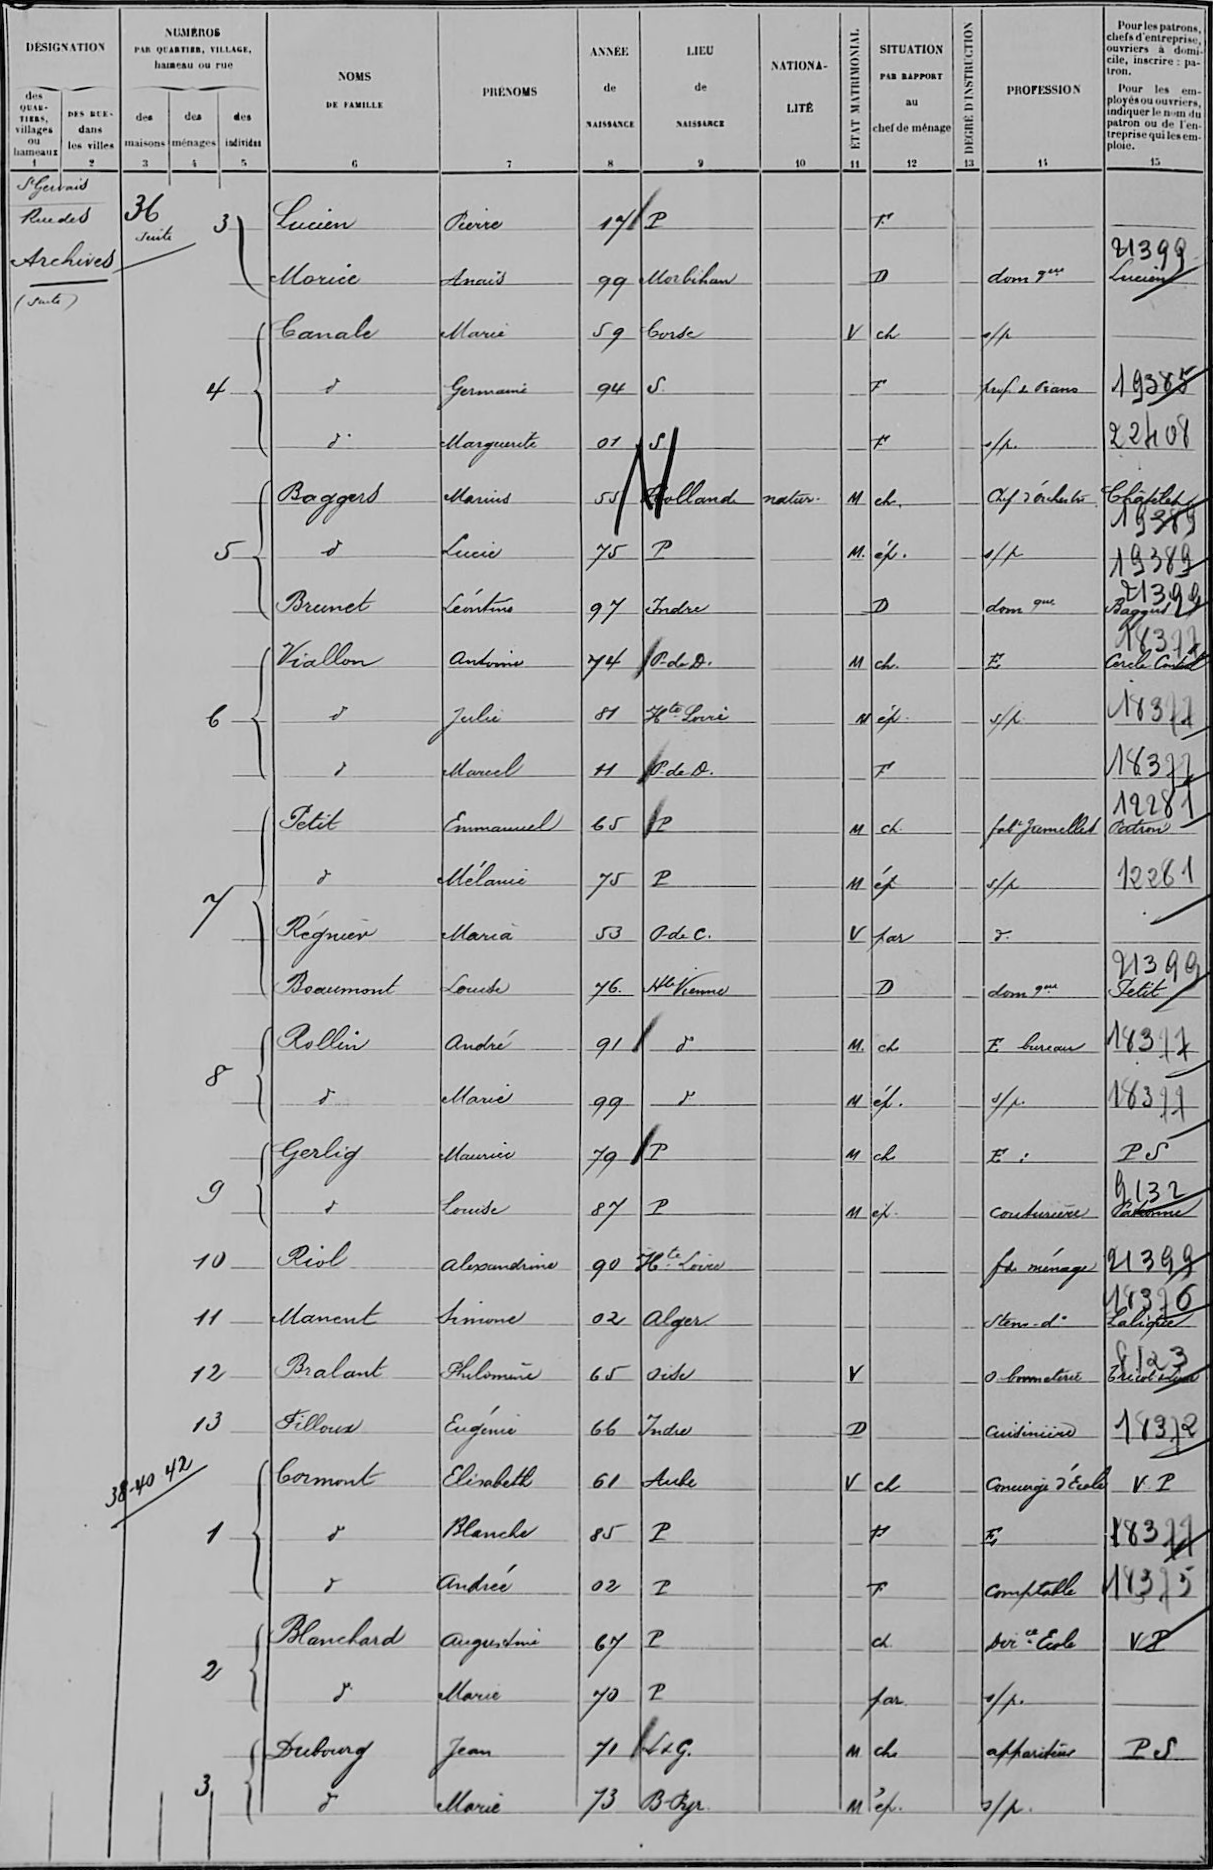Lucien/Pierre/17/P/¤/¤/F/¤/¤/¤
Morin/Anais/99/Morbihan/¤/¤/D/¤/domque/Lucien?21399
Canale/Marie/59/Corse/¤/V/ch/¤/s:p/¤
d/germaine/94/S /¤/¤/F/¤/prof de piano/19385
d/Marguerite/01/S/¤/¤/F/¤/s:p/22408
Baggers/Marius/55/Hollande/natur /M/ch/¤/Chef dorchestre/Châtelet!19389
d/Lucie/75/P/¤/M /ép/¤/s:p/19389
Brunet/léontine/97/Indre/¤/¤/D/¤/domque/Baggers?21399
Viallon/antoine/74/P de D /¤/M/ch /¤/E/Cercle Courtal?18377
d/Julie/81/Hte Loire/¤/M/ép/¤/s:p/18377
d/Marcel/11/P de D /¤/¤/F/¤/¤/18377
Petit/Emmanuel/65/P/¤/M/ch /¤/fabt jumelles/Patron12281
d/Mélanie/75/P/¤/M/ép/¤/s:p/12281
Régnier/Maria/53/P de C /¤/V/par/¤/d /¤
Beaumont/Louise/76/Hte Vienne/¤/¤/D/¤/domque/Petit?21399
Rollin/André/91/d/¤/M/ch/¤/E bureau/18377
d/Marie/99/d/¤/M/ép /¤/s:p/18377
Gerlig/Maurice/79/P/¤/M/ch/¤/E/P S
d/Louise/87/P/¤/M/ép/¤/couturière/Patronne9132
Riol/Alexandrine/90/Hte Loire/¤/¤/¤/¤/F de ménage/21399
Manent/Simone/02/Alger/¤/¤/¤/¤/Steno d°/Lalique?18376
Bralant/Philomène/65/oise/¤/V/¤/¤/o bonneterie/tricot adim?8123
Filloux/Eugénie/66/Indre/¤/D/¤/¤/cuisinière/18372
Cormont/Elisabeth/61/Aube/¤/V/Ch/¤/Concierge d'école/V P
d/Blanche/85/P/¤/¤/F/¤/E/18377
d/Andrée/02/P/¤/¤/F/¤/Comptable/18375
Blanchard/Augustine/67/P/¤/¤/ch/¤/Dirce Ecole/VP
d/Marie/80/P/¤/¤/par/¤/s:p/¤
Dubourg/Jean/71/L & G/¤/M/ch /¤/appariteur/PS
d/Marie/73/B Pyr/¤/M/ép/¤/s:p/¤
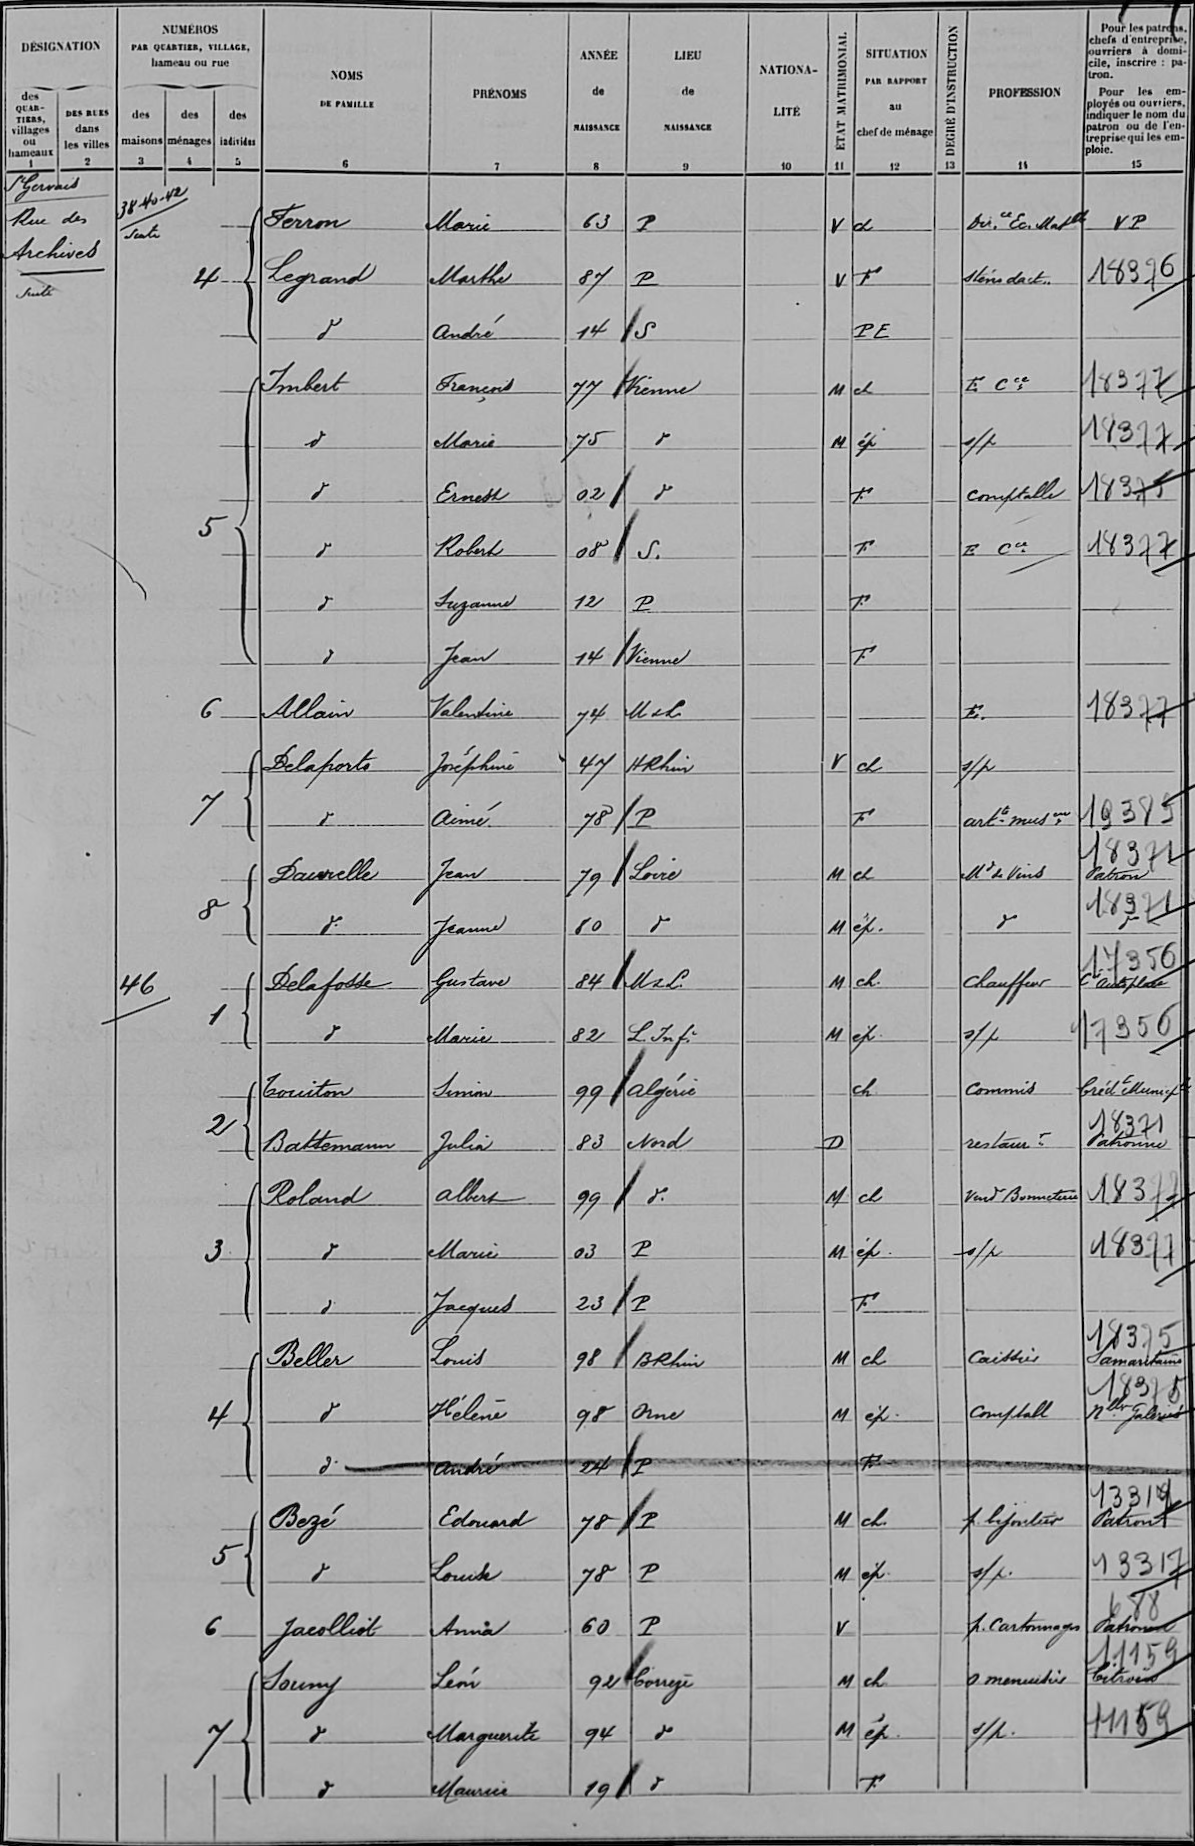Ferron/Marie/63/P/¤/V/d/¤/Dirce Ec Maelle/VP
Legrand/Marthe/87/P/¤/V/F/¤/sténo dact/18376
d/André/14/S/¤/¤/PE/¤/¤/¤
Imbert/François/77/Vienne/¤/M/ch/¤/E Cce/18377
d/Marie/75/d/¤/M/ép/¤/s:p/18377
d/Ernest/02/d/¤/¤/F/¤/comptable/18375
d/Robert/08/S /¤/¤/F/¤/E Cce/18377
d/Suzanne/12/P/¤/¤/F/¤/¤/¤
d/Jean/14/Vienne/¤/¤/F/¤/¤/¤
Allain/Valentine/74/M & L/¤/¤/¤/¤/E/18377
Delaporte/Joséphine/47/H Rhin/¤/V/ch/¤/s:p/¤
d/Aimé/78/P/¤/¤/F/¤/artte musenne/19389
Daurelle/Jean/79/Loire/¤/M/ch/¤/Md de Vins/Patron?18371
d/Jeanne/80/d/¤/M/ép /¤/d/d?18371
Delafosse/Gustave/84/M & L/¤/M/ch /¤/Chauffeur/C autoplace?17356
d/Marie/82/L Infe/¤/M/ép/¤/s:p/17356
Touiton/Simon/99/algérie/¤/¤/ch/¤/commis/Créd Municip
Battemann/Julia/83/Nord/¤/D/¤/¤/restaur/Patronne?18371
Roland/albert/99/d/¤/M/ch/¤/Vend Bonneterie/18377
d/Marie/03/P/¤/M/ép/¤/s:p/18377
d/Jacques/23/P/¤/¤/F/¤/¤/¤
Beller/Louis/98/B Rhin/¤/M/ch/¤/Caissier/Samaritaine?18375
d/Hélène/98/Orne/¤/M/ép /¤/Comptable/Nlle galeries?18378
d/andré/24/P/¤/¤/F/¤/¤/¤
Bezé/Edouard/78/P/¤/M/ch /¤/p bijoutier/Patron?13319
d/Louise/78/P/¤/M/ép/¤/s:p/13317
Jacolliot/Anna/60/P/¤/v/¤/¤/p cartonnage/Patronne?688
Souny/Léon/92/Corrèze/¤/M/ch/¤/o menuisier/Citroën?11159
d/Marguerie/94/d/¤/M/ép/¤/s:p/11159
d/Maurice/19/d/¤/¤/F/¤/¤/¤